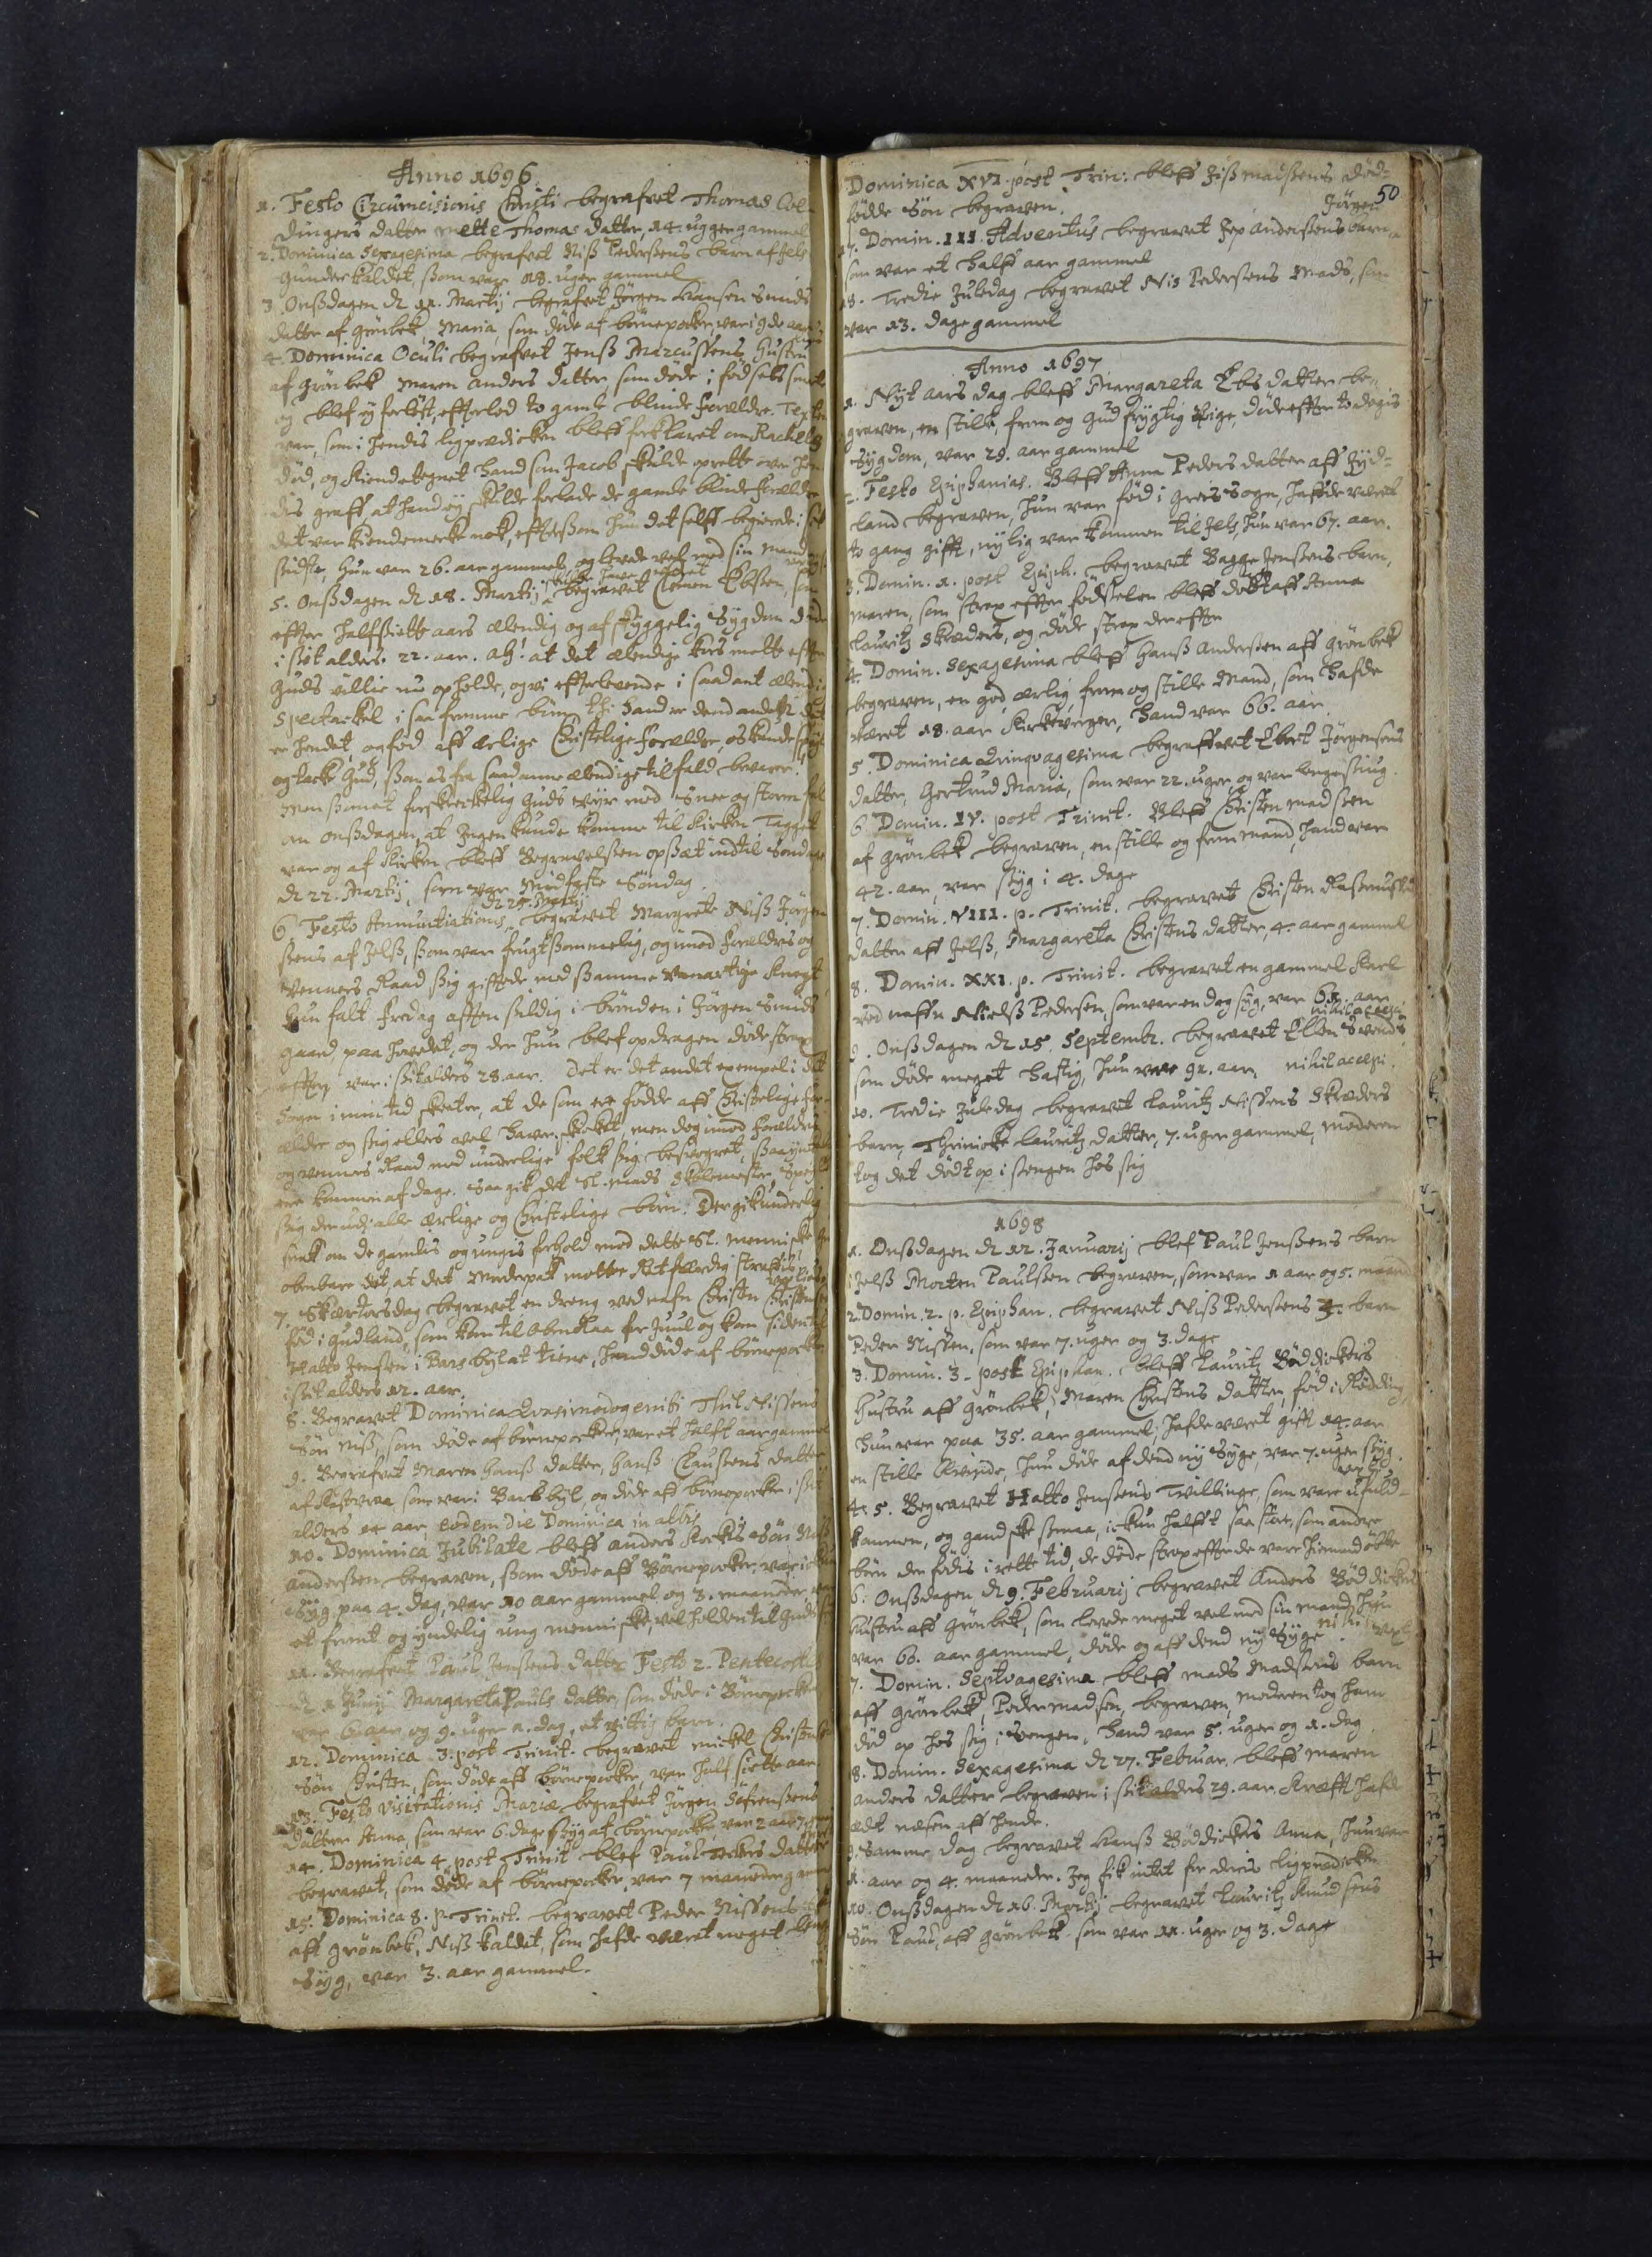Anno 1696
1. Festo Circumcisionis Christi begrafvet Thomas Col¬
dingers dater Mette Thomas Datter, 14. Ugger gammel
2. Dominica Sexagesima begrafvet Niss Pederssens barn aff Jels
Gunder kaldet, ssom var 18. uger gammel.
3. Onssdagen d 11 Martij begrafvet Jørgen Hansen Smids
Datter af Grønbek, Maria, som døde af børnepocker, var i 9de aar.
4. Dominica Oculi begrafvet Jenss Marcussens hustru
af Grønbek Maren Anders Datter, som døde i fødsels smerte
og blef ey forløst, effterlod to gamle blinde forældre. Texten
var, som i hendis ligprædiken bleff forklaret om Rachels
død, og kiendetegnet hand som Jacob skulde oprette over hen
dis graff, at hand ey skulde forlade de gamle blinde forældre
det var Kiendemerke nok, efftersom hun det selff begierede i sit
sidste, hun var 26. aar gammel, og levde vel med sin mand
skulde have været
5. Onssdagen d 18. Martij begravet Clemen Ebsen, som
efter halfsiette aars ælendig og afskyggelig Sygdom døde
i ssit alders 22. aar. Ah! at det ælendige kors motte efter
Guds villie nu opholde, og vi efterlevende i saadant ælendig
spectackel i saa fromme bøn## thi hand er dend ande## det##
er hendet, og fød aff ærlige Christelige forældre, os kende
og l##ke Gud, ssom os fra saadanne ælendige tilfald bevare!
men ssom et forskierkelig Guds vijr med sne og stormfal 
om onssdagen, at ingen kunde komme til Kirken Tagget
var og af Kirken blest Begravelsen opssæt indtil Søndag
d 22 Martij, som var Midfaste Søndag.
d 25. Martij
6. Festo Annuntiations begravet Margrete Niss Jørgen
ssens af Jelss, ssom var frugtssommelig, og imod forældrenes og
venners Raad ssig giftede med ssamme vanartige Knegt
hun falt fredag aften ssidlig i brønden i Jørgen Smids
gaard paa hovedet, og der hun blef opdragen døde strax
effter var i ssit alders 28. aar. Det er det andet exsempel i det
Sogn i min tid skete##, at de som ere fødde aff Christelige for¬
ældre og ssig ellers vel have skicket, men dog imod forældres
og venners Raad med underlige folk sig besvogret, ssaa
ere kommen af dage. Saa gik det Sl. Mads Skolmester
ssig den udi alle ærlige og Christelige bøn. Der gik underlig
snak om de gamles og unges forhold mod dette Sl. Menniske
obenbare det##, at det Morderpak## motte Retfærdig straffis
v## livet##
7. Skærtorsdag begravet en dreng ved nafn Christen Christensen
fød i Gudland, som kom til Obenraa før Juul og kom siden til
Hatto Jenssen i Barsbyl at tiene, hand døde aff børnepocker
i sit alders 12. aar.
8. Begravet Dominica Qvasimodogerniti Thil Nissens
Søn Niss, som døde af børnepocker, var et halft aar gammel
9. Begrafvet Maren Hanss Datter, Hanss Claussens datter
af Kastvraa, som var i Barsbyl og døde aff børnepocker i ssit
alders 14 aar, eodem die dominica in
10. Dominica Jubilate bleff Anders Kockis Søn Niss
Andersen begraven, ssom døde aff Børnepocker, var ickun
syg paa 4. Dag, var 10 aar gammel og 3. maaneder var
et fromt og yndelig## ung menniske, vel holden til Guds ##
11. Begrafvent Paul Jenssens datter Festo 2. Pentecostes
d 1 Junij Margareta Pouls datter, som døde i børnepocker
var 6 aar og 9. uger 1 dag, et vittig barn.
12. Dominica 3. post Trinit. begravet Mickel Christensens
Søn Christen, som døde aff børnepocker, var half siette aar.
13. Festo Visitationis Mariæ begrafvet Jørgen Søfrenssens
Datter Anna, som var 6. dage ssyg af børnepocker, var 2 aar 7
Kirs##
14. Dominica 4. post Trinit blefff Paul Teckers Datter
begravet, som døde af børnepocker, var 7 maaneder gammel
15. Dominica 8. p. Trinit. begravet Peder Nissens
aff Grønbek, Niss kaldet, som hafde veret noget lenge
syg, var 3. aar gammel.
50
16. Dominica XVI post Trin: bleff Jiss Madssens død
fødde Søn begraven.
Jørgen
17. Domin. III Adventus begravet Jep Anderssens barn
som var et halff aar gammel
18. Tredie Juledag begravet Nis Pedersens Mads, som
var 13. dage gammel
Anno 1697
1. Nyt aars dag bleff Margareta Ebs Datter be
graven, en stille, from og gud frygtig Pige, døde effter to dages
Sygdom, var 29. aar gammel
2. Festo Epiphanias. Bleff Anna Peders Datter aff Jyd¬
land begraven, hun var fød i Greis Sogn, hafde veret
to gang gifft, nylig var kommen til Jels, hun var 67. aar.
3. Domin. 1. post Epiph. begravet Bagge Jenssens barn,
Maren, som strax effter fødselen bleff døbt aff Anna
Lauritz Skræders, og døde strax dereffter.
4. Domin. Sexagesima bleff Hanss Anderssen aff Grønbek
begraven, en god, ærlig, from og stille Mand, som hafde
været 18. aar Kirkeverger, hand var 66. aar.
5. Dominica Qvinquagesima begraffvet Ebert Jørgensens
datter, Gertrud Maria, som var 22. uger og var lenge siug.
6. Domin. IV. Post Trinit. Blef Christen Madssen
af Grønbek begraven, en stille og from mand, han var
42. aar, var ssiug i 4. dage
7. Domin. VIII. p. Trinit. begravet Christen Rassmussens
datter aff Jelss, Margareta Christens datter, 4. aar gammel
8. Domin. XXI. p. Trinit. begravet en gammel Karl
ved naffn Nielss Pedersen, som var en dag syg, var 61. aar
Nihil accepi
9. Onsdagen d 15. Septembr. begravet Ellen Svends
som døde meget hastig, hun var 92. aar. Nihil accepi.
10. Tredie Juledag begravet Lauritz Nissens Skræders
barn, Thrinicke Lauritz datter, 7. uger gammel, moderen
tog det dødt op i sengen hos sig
1698
1. Onsdagen d 12 Januarij blef Paul Jenssens barn
i Jelss Morten Paulssen begraven, som var 1 aar og 5. maaned
2. Domin. 2. p. Epiphan. begravet Niss Pedersens 4. barn
Peder Nissen, som var 7. uger og 3. dage
3. Domin. 3. post Epiphan. bleff Lauritz Bøddickers
Hustru aff Grønbek, Maren Christens datter, fød i Rødding,
hun var paa 35. aar gammel, hafde været gift 14. aar
en stille Qvinde, hun døde af dend ny Syge, var 7. uger ssyg.
##
4. 5. Begravet Hatto Jenssens Tvilinge, som vare ufuld¬
kommen, og gandske ssmaa, ickun halfft saa stor, som andre
børn der fødis i rette tid, de døde strax effter de var hiemedøbte.
6. Onssdagen d 9. Februarij begravet Anders Bøddickers
Hustru aff Grønbek, som levede meget vel med sin mand, hun
var 60. aar gammel, døde og aff dend ny Syge. Nihil accepi
7. Domin Septuagesima bleff Mads Madssens barn
aff Grønbek, Peder Madsen, begraven, moderen tog ham
død op hos sig i sengen. Hand var 5. uger og 1. dag
8. Domin. Sexagesima d 27. Februar. Bleff Maren
Anders Datter begraven i ssit alders 29. aar. Kræfft hafde
ædt næsen aff hende.
9. Samme dag begravet Hanss Bøddickers Anna, hun var
1. aar og 4. maaneder. Jeg fik intet for dend Ligprædicken
10. Onssdagen d 16. Martij begravet Lauritz Knudsens
Søn Paul aff Grønbeck, som var 11. uger og 3. dage